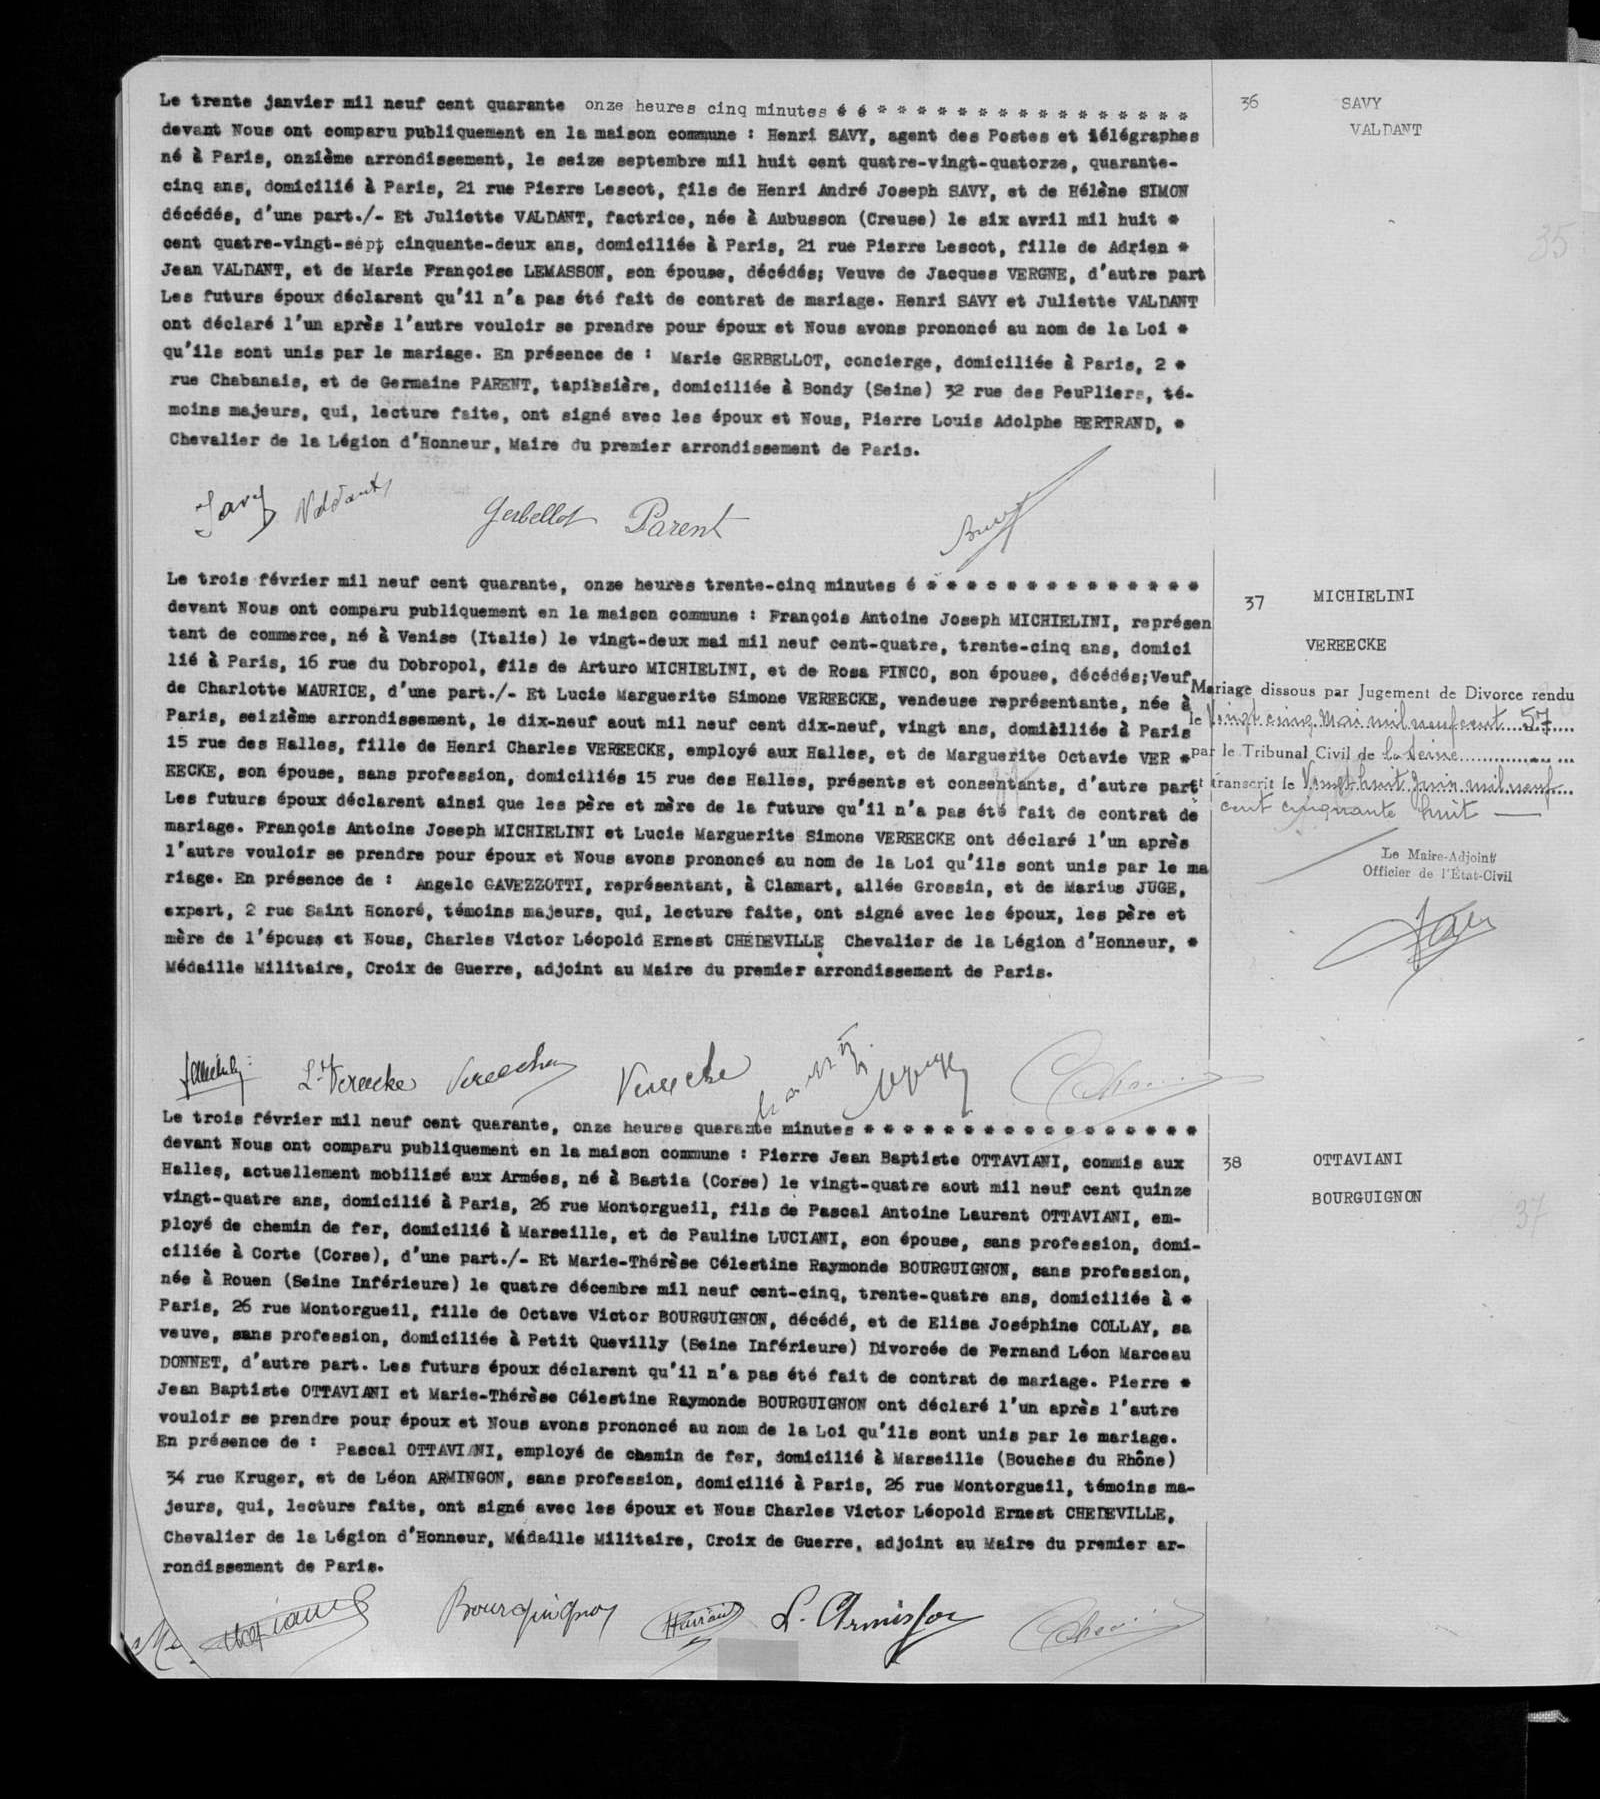Le trente janvier mil neuf cent quarante onze heures cinq minutes ****
devant Nous ont comparu publiquement en la maison commune: Henri SAVY, agent des Postes et télégraphes
né à Paris, onzième arrondissement, le seize septembre mil huit cent quatre-vingt-quatorze, quarante-
cinq ans, domicilié à Paris, 21 rue Pierre Lescot, fils de Henri André Joseph SAVY, et de Hélène SIMON
décédés, d'une part ./-Et Juliette VALDANT, factrice, née à Aubusson (Creuse) le six avril mil huit *
cent quatre-vingt-sept cinquante-deux ans, domiciliée à Paris, 21 rue Pierre Lescot, fille de Adrien *
Jean VALDANT, et de Marie Françoise LEMASSON, son épouse, décédés; Veuve de Jacques VERGNE, d'autre part
les futurs époux déclarent qu'il n'a pas été fait de contrat de mariage. Henri SAVY et Juliette VALDANT
ont déclaré l'un après l'autre vouloir se prendre pou époux et Nous avons prononcé au nom de la Loi *
qu'ils sont unis par le mariage. En présence de: Marie GERBELLOT, concierge, domiciliée à Paris, 2 *
rue Chabanais, et de Germaine PARENT, tapissière, domiciliée à Bondy (Seine) 32 rue des Peupliers, té-
moins majeurs, qui, lecture faite, ont signé avec les époux et Nous, Pierre Louis Adolphe BERTRAND, *
Chevalier de la Légion d'Honneur, Maire du premier arrondissement de Paris.
Le trois février mil neuf cent quarante, onze heures trente-cinq minute é ****
devant Nous ont comparu publiquement en la maison commune: François Antoine Joseph MICHIELINI, représen
tant de commerce, né à Venise (Italie) le vingt-deux mai mil neuf cent-quatre, trente-cinq ans, domici
lié à Paris, 16 rue du Dobropol, fils de Arturo MICHIELINI, et de Rosa FINCO, son épouse, décédés; Veuf
de Charlotte MAURICE, d'une part ./-Et Lucie Marguerite Simone VEREECKE, vendeuse représentante, née à
Paris, seizième arrondissement, le dix-neuf aout mil neuf cent dix-neuf, vingt ans, domiciliée à Paris
15 rue des Halles, fille de Henri Charles VERRECKE, employé aux Halles, et de Marguerite Octavie VER *
KECKE, son épouse, sans profession, domiciliés 15 rue des Halles, présents et consentants, d'autre part
Les futurs époux déclarent ainsi que les père et mè de la future qu'il n'a pas été fait de contrat de
mariage. François Antoine Joseph MICHIELINI et Lucie Marguerite Simone VEREECKE ont déclaré l'un après
l'autre vouloir se prendre pour époux et Nous avons prononcé au nom de la Loi qu'ils sont unis par le ma
riage. En présence de: Angele GAVEZZOTTI, représentant, à Clamart, allée Grossin, et de Marius JUGE,
expert, 2 rue saint Honoré, témoins majeurs, qui, lecture faite, ont signé avec les époux, les père et
mère de l'épouse et Nous, Charles Victor Léopold Ernest CHELEVILLE Chevalier de la Légion d'Honneur, *
Médaille Militaire, Croix de Guerre, adjoint au Maire du premier arrondissement de Paris.
Le trois février mil neuf cent quarante, onze heures quarante minutes ****
devant Nous ont comparu publiquement en la maison commune: Pierre Jean Baptiste OTTAVIANI, commis
aux Halles, actuellement mobilisé aux Armées, né à Bastia (Corse) le vingt-quatre aout mil neuf cent quinze
vingt-quatre ans, domicilié à Paris, 26 rue Montorgueil, fils de Pascal Antoine Laurent OTTAVIANI, em-
ployé de chemin de fer, domicilié à Marseille, et de Pauline LUCIANI, son épouse, sans profession, domi-
ciliée à Corte (Corse), d'une part ./-Et Marie-Thérèse Célestine Raymonde BOURGUIGNON, sans profession,
née à Rouen (Seine Inférieure) le quatre décembre mil neuf cent-cinq, trentre-quatre ans, domiciliée à *
Paris, 26 rue Montorgueil, fille de Octave Victor BOURGUIGNON, décédé, et de Elisa Joséphine COLLAY, sa
veuve, sans profession, domiciliée à Petit Quevilly (Seine Inférieure) Divorcée de Fernand Léon Marceau
DONNET, d'autre part. Les futurs époux déclarent qu'il n'a pas été fait de contrat de mariage. Pierre *
Jean Baptiste OTTAVIANI et Marie-Thérèse Célestine Raymonde BOURGUIGNON ont déclaré l'un après l'autre
vouloir se prendre pour époux et Nous avons prononcé au nom de la Loi qu'ils sont unis par le mariage.
En présence de: Pascal OTTAVIANI, employé de chemin de fer, domicilié à Marseille (Bouches du Rhône)
34 rue Kruger, et de Léon ARMINGON, sans profession, domicilié à Paris, 26 rue Montorgeuil, témoins ma-
jeurs, qui, lecture faite, ont signé avec les époux et Nous Charles Victor Léopold Ernest CHELEVILLE,
Chevalier de la Légion d'Honneur, Médailler Militaire, Croix de Guerre, adjoint au Maire du premier ar-
bondissement de Paris.
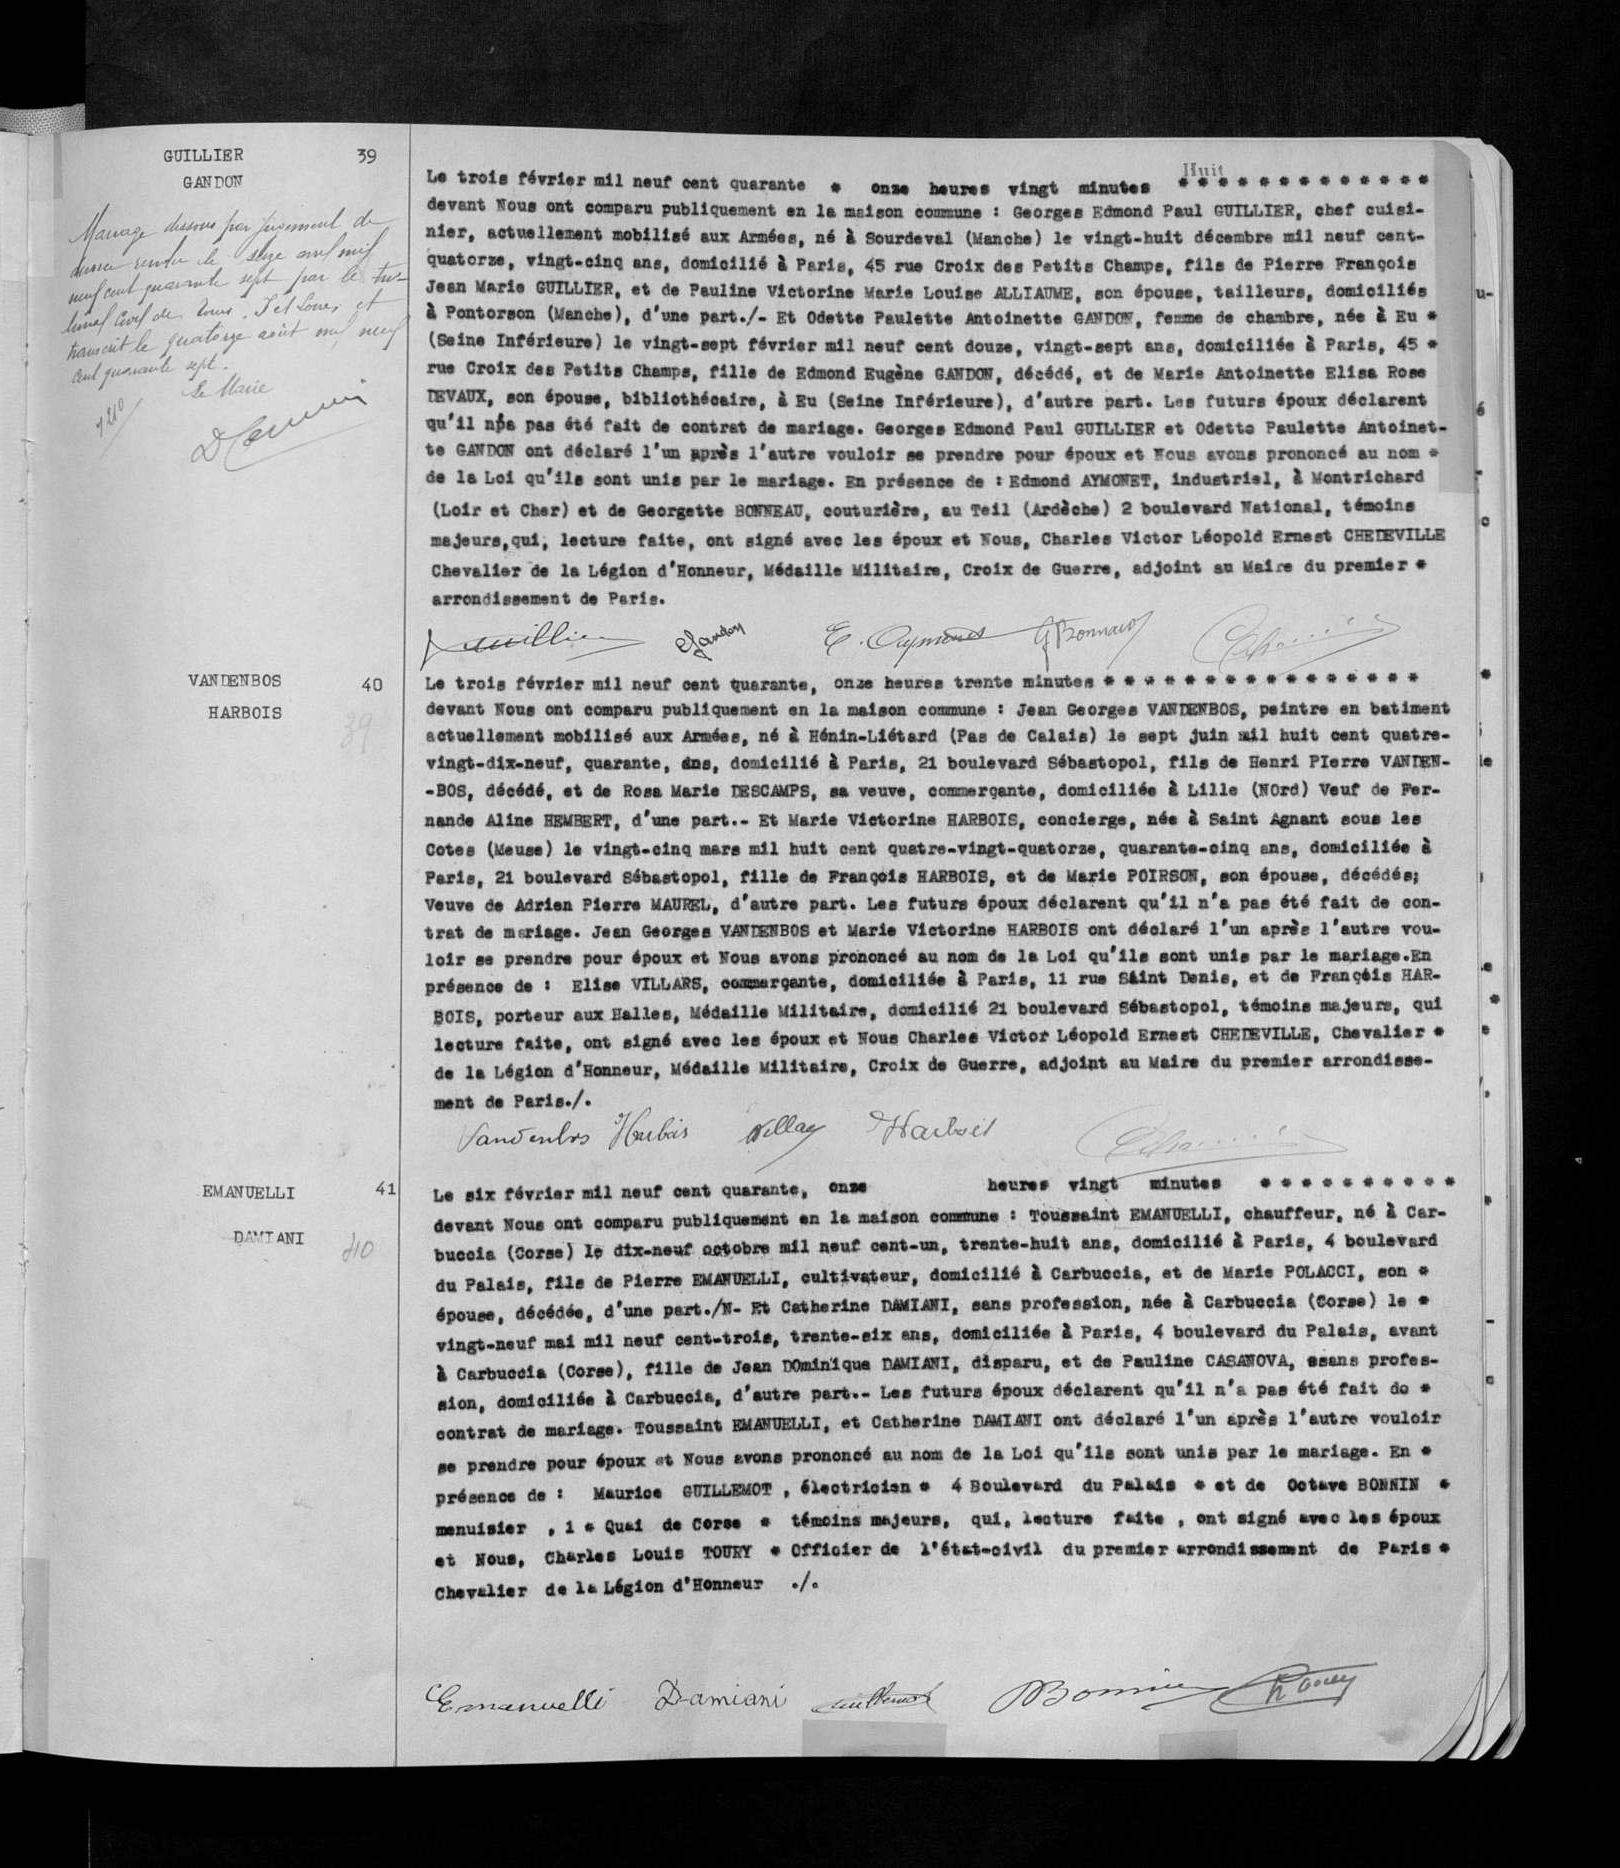Le trois février mil neuf cent quarante * onze heures vingt minutes ****
devant Nous ont comparu publiquement en la maison commune: Georges Edmond Paul GUILLIER, chef cuisi-
nier, actuellement mobilisé aux Armées, né à Sourdeval (Manche) le vingt-huit décembre mil neuf cent-
quatorze, vingt-cinq ans, domicilié à Paris, 45 rue Croix des Petits Champs, fils de Pierre François
Jean Marie GUILLIER, et de Pauline Victorine Marie Louise ALLIAUME, son épouse, tailleurs, domiciliés
à Pontorson (Manche), d'une part ./-Et Odette Paulette Antoinette GANDON, femme de chambre, née à Eu *
(Seine Inférieure) le vingt-sept février mil neuf cent douze, vingt-sept ans, domiciliée à Paris, 45 *
rue Croix des Petits Champs, fille de Edmond Eugène GANDON, décédé, et de Marie Antoinette Elisa Rose
DEVAUX, son épouse, bibliothécaire, à Eu (Seine Inférieure), d'autre part. Les futurs époux déclarent
qu'il npa été fait de contrat de mariage. Georges Edmond Paul GUILLIER et Odette Paulette Antoinet-
te GANDON ont déclaré l'un après l'autre vouloir se prendre pour époux et Nous avons prononcé au nom *
de la Loi qu'ils sont unis par le mariage. En présence de: Edmond AYMONET, industriel, à Montrichard
(Loir et Cher) et de Georgette BONNEAU, couturière, au Teil (Ardèche) 2 boulevard National, témoins
majeurs, qui, lecture faite, ont signé avec les époux et Nous, Charles Victor Léopold Ernest CHELEVILLE
Chevalier de la Légion d'Honneur, Médaille Militaire, Croix de Guerre, adjoint au Maire du premier *
arrondissement de Paris
Le trois février mil neuf cent quarante, onze heures trente minutes ****
devant Nous ont comparu publiquement en la maison commune: Jean Georges VANDENBOS, peintre en bâtiment
actuellement mobilisé aux Armées, né à Hénin-Liétard (Pas de Calais) le sept juin mil huit cent quatre-
vingt-dix-neuf, quarante, ans, domicilié à Paris, 21 boulevard Sébastopol, fils de Henri Pierre VANIEN-
-BOS, décédé, et de Rosa Marie DESCAMPS, sa veuve, commerçante domicilié à Lille (NOrd) Veuf de Fer-
lande Aline HEMBERT, d'une part .-Et Marie Victorine HARBOIS, concierge, née à Saint Agnant sous les
Cotes (Meuse) le vingt-cinq mars mil huit cent quatre-vingt-quatorze, quarante-cinq ans, domiciliée à
Paris, 21 boulevard Sébastopol, fille de François HARBOIS, et de Marie POIRSON, son épouse, décédés;
Veuve de Adrian Pierre MAUREL, d'autre part. Les futurs époux déclarent qu'il n'a pas été fait de con-
trat de mariage. Jean Georges VANDENBOS et Marie Victorine HARBOIS ont déclaré l'un après l'autre vou-
loir se prendre pour époux et Nous avons prononcé au nom de la Loi qu'ils sont unis par le mariage. En
présence de: Elise VILLARS, commerçante, domiciliée à Paris, 11 rue Saint Denis, et de François HAR-
BOIS, porteur aux Halles, Médaille Militaire, domicilié 21 boulevard Sébastopol, témoins majeurs, qui
lecture faite, ont signé avec les époux et Nous Charles Victor Léopold Ernest CHELEVILLE, Chevalier *
de la Légion d'Honneur, Médaille Militaire, Croix de Guerre, adjoint au Maire du premier arrondisse-
ment de Paris ./.
Le six février mil neuf cent quarante, onze heures vingt minutes ****
devant Nous ont comparu publiquement en la maison commune: Toussaint EMANUELLI, chauffeur, né à Car-
buccia (Corse) le dix-neuf octobre mil neuf cent-un, trente huit ans, domicilié à Paris, 4 boulevard
du Palais, fils de Pierre EMANUELLI, cultivateur, domicilié à Carbuccia, et de Marie POLACCI, son *
épouse, décédée, d'une part ./ N-Et Catherine DAMIANI, sans profession, née à Carbuccia (Corse) le *
vingt-neuf mai mil neuf cent-trois, trente-six ans, domiciliée à Paris, 4 boulevard du Palais, avant
à Carbuccia (Corse), fille de Jean Dominique DAMIANI, disparu, et de Pauline CASANOVA, ssans profes-
sion, domiciliée à Carbuccia, d'autre part .-Les futurs époux déclarent qu'il n'a pas été fait de *
contrat de mariage. Toussaint EMANUELLI, et Catherine DAMIANI ont déclaré l'un après l'autre vouloir
se prendre pour époux et Nous avons prononcé au nom de la Loi qu'ils sont unis par le mariage. En *
présence de: Maurice GUILLEMOT, électricien * 4 Boulevard du Palais * et de Octave BONNIN *
menuisier, 1 * Quai de Corse * témoins majeurs, qui, lecture faite, ont signé avec les époux
et Nous, Charles Louis TOURY * Officier de l'état-civil du premier arrondissement de Paris *
Chevalier de la Légion d'Honneur ./.